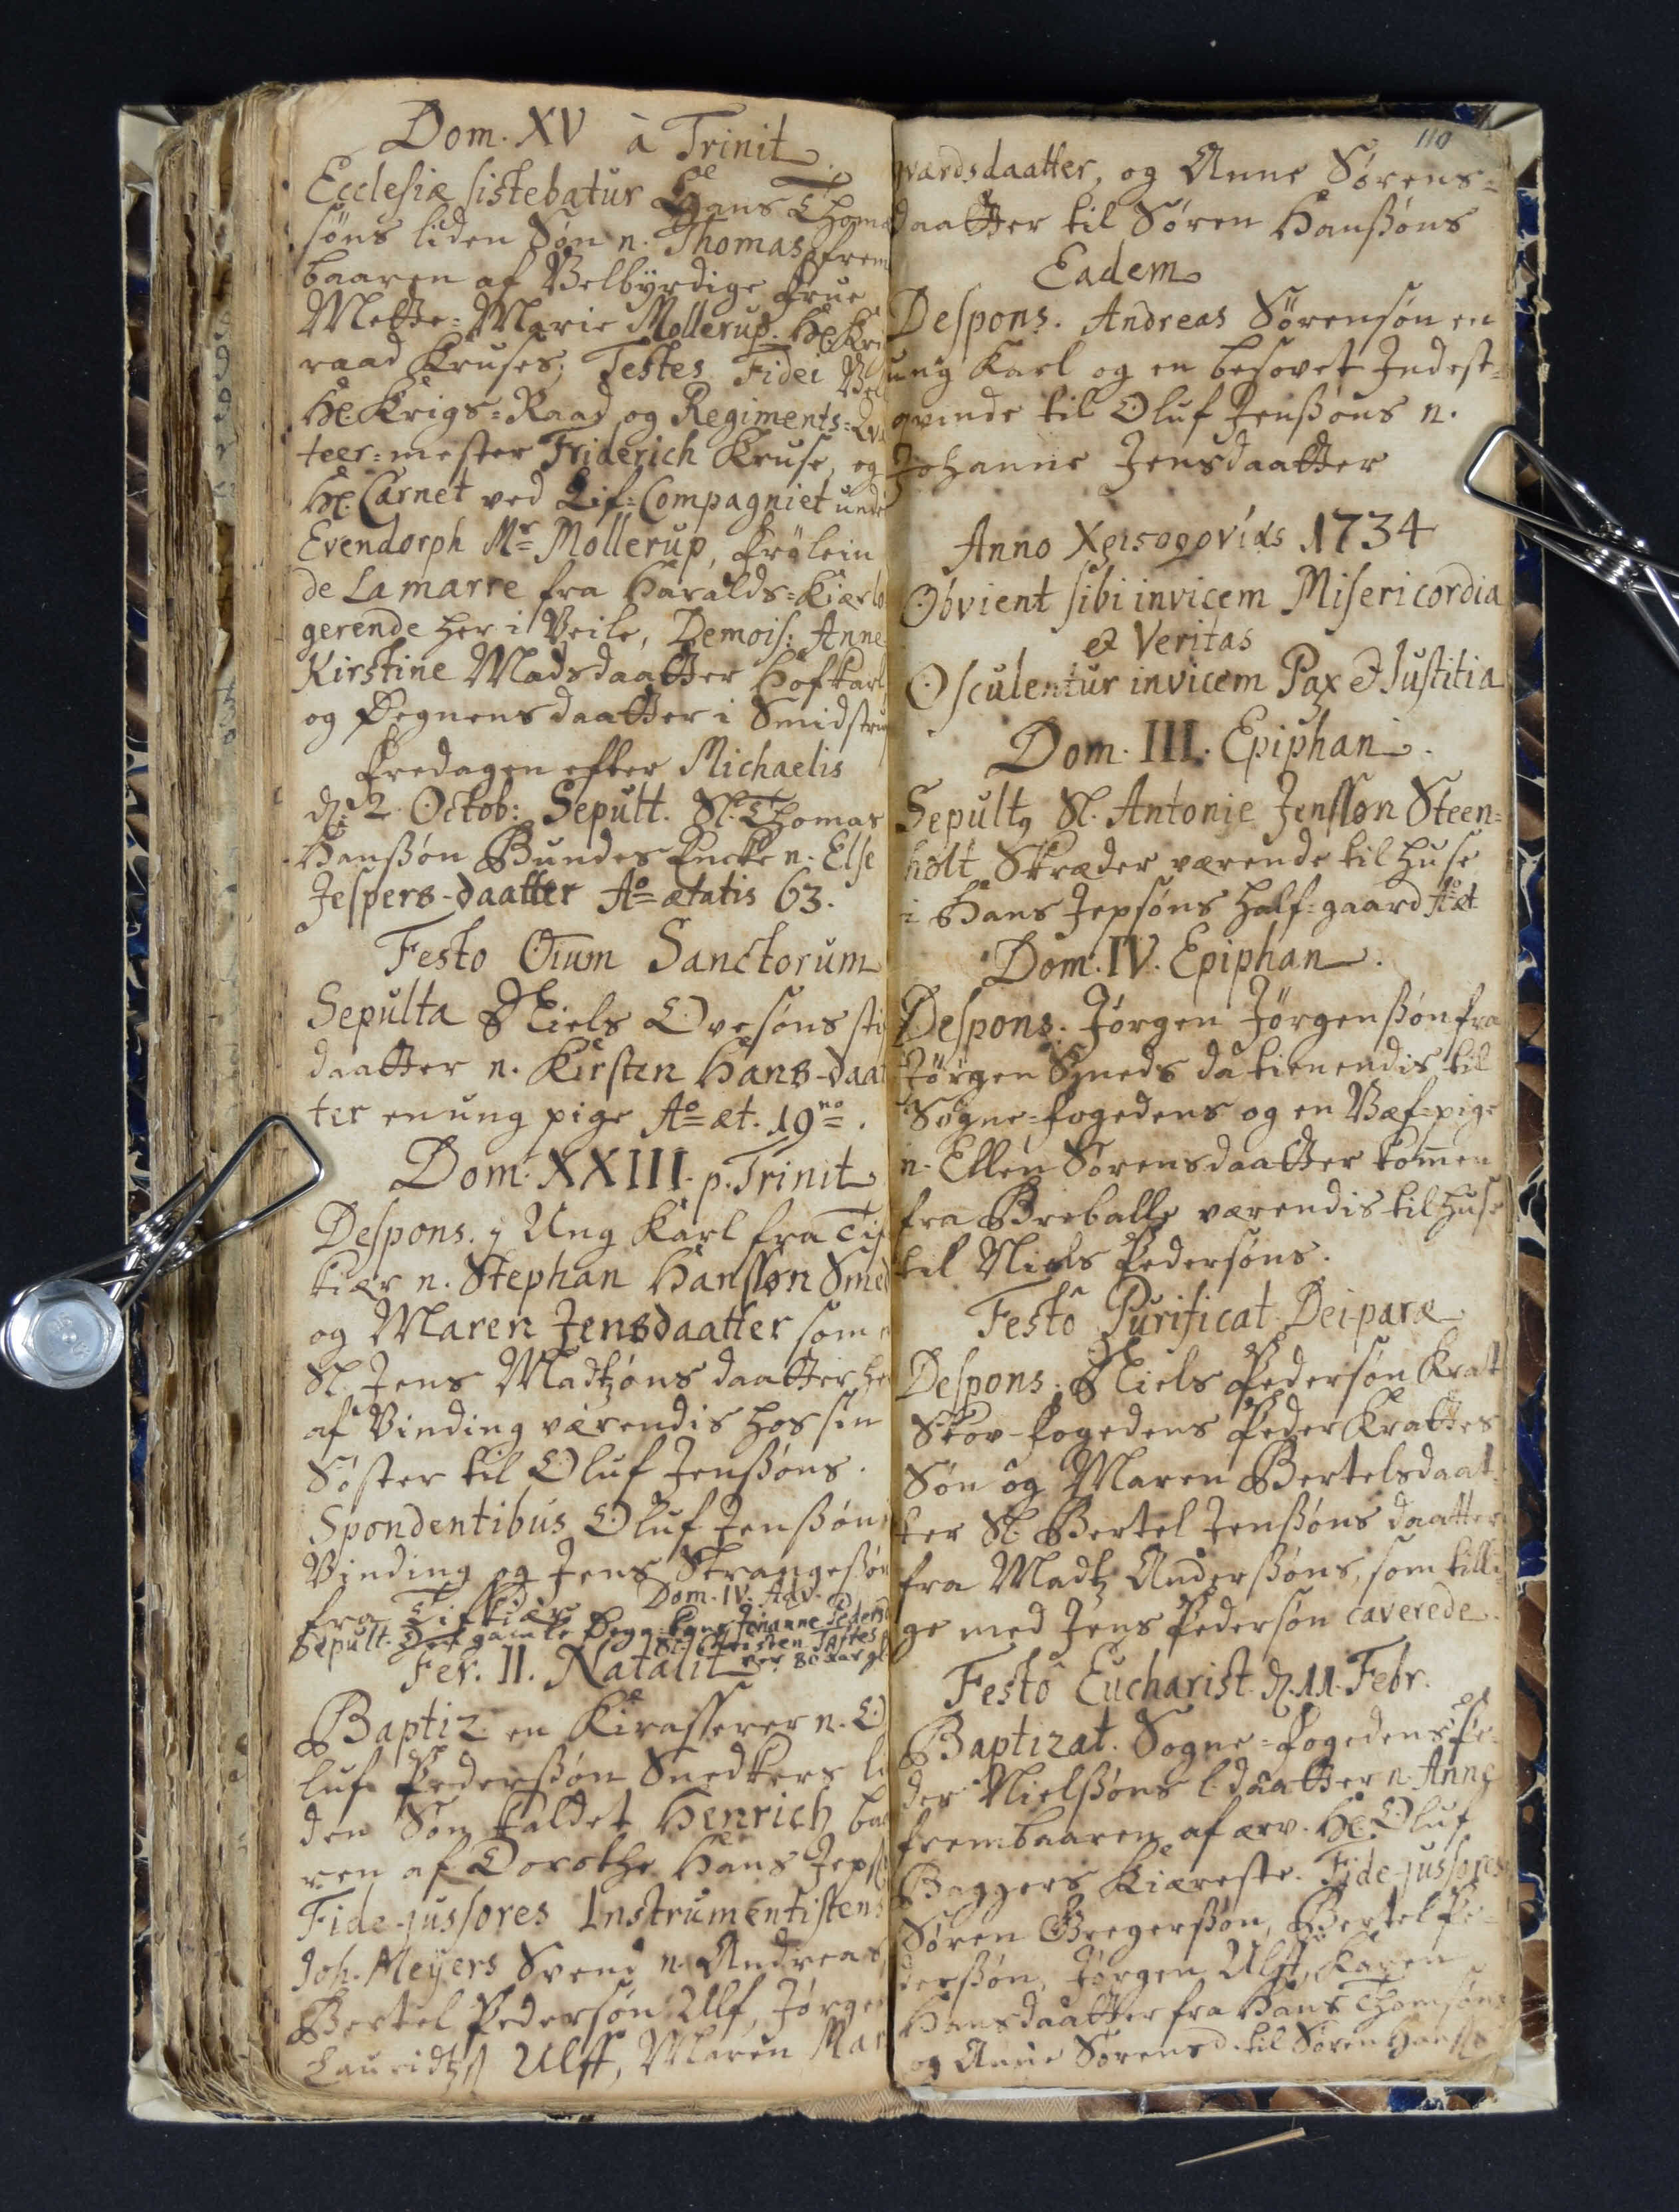Dom. XV a Trinit
Ecclesiæ sistebatur Hans Thomæ
søns liden Søn n. Thomas, frem
baaren af Velbyrdige Frue
Mette=Marie Mollerup Hl Kri  
raad Kruses; Testes Fidei Vel  
Hl Krigs=Raad og Regiments=Qvi  
teer=mester Friderich Kruse, og
Hl Carnet ved Lif=Compagniet unde  
Evendorph Mr Mollerup, Frølein
de la marre fra Haralds=Kiær lo
gerende her i Veile, Demois: Anne
Kirstine Madsdaatter Hofkarl
og Degnens daatter i Smidstrup
Fredagen efter Michaelis
d. 2. Octob: Sepult. Sl. Thomas
Hanssøn Bundes Encke n. Else
Jespers-daatter Ao ætatis 63.
Festo Oium Sanctorum
Sepulta Niels Ovesøns stif
daatter n. Kirsten Hansdaat
ter en ung pige Ao æt. 19no.
Dom. XXIII. p. Trinit
Despons. 1 Ung Karl fra Tif
kiær n. Stephan Hanssøn Smed
og Maren Jensdaatter som
Sl. Jens Madtzøns daatter her  
af Vinding værendis hos sin
Søster til Oluf Jenssøns.
Spondentibus Oluf Jenssøn i
Vinding og Jens Strangessøn
fra Tifkiær.
Dom. IV. Adv.
Sepult. Den gamle Degn=Kone Johanne Pedersd
Sl. Christen Taftes
var 80 aar gl.
Fer. II. Natalit
Baptiz. en Kirasserer n. O
luf Pederssøn Snedkers li
den Søn kaldet Henrich baa  
ren af Dorothe Hans Jeps
Fide-jussores Instrumentistens
Joh. Meyers Svend n. Andreas
Bertel Pedersøn Ulf, Jørgen
Lauridtzs Ulff, Maren Mar
110
qvardsdaatter, og Anne Sørens¬
daatter til Søren Hanssøns
Eadem
Despons. Andreas Sørensøn en
ung Karl og en besovet Indest¬
qvinde til Oluf Jenssøns n.
Johanne Jensdaatter
Anno X##ovixs 1734
obvient sibi invicem Misericordia
et Veritas
Osculentur invicem Pax et Justitia
Dom. III. Epiphan
Sepultq Sl. Antonie Jenssøn Steen¬
holt Skræder værende til huse
i Hans Jepsøns half=gaard Ao æt.
Dom. IV. Epiphan
Despons. Jørgen Jørgenssøn fra
Jørgen Smeds da tienendis til
Sogne=fogedens og en Væf=pige
n. Ellen Sørensdaatter kommen
fra Breballe værendis til huse
til Niels Pedersøns
Festo Purificat Dei-paræ
Despons. Niels Pedersøn Krat
Skov-fogedens Peder Krattes
søn og Maren Bertelsdaat¬
ter Sl. Bertel Jenssøns daatter
fra Madtz Anderssøns som tilli
ge med Jens Pedersøn caverede.
Festo Eucharist d. 11. Febr.
Baptizat. Sogne=Fogedens Pe¬
der Nielssøns l. daatter n. Anne,
frembaaren af ærv. Hl Oluf
Baggers Kiæreste. Fide-jussores
Søren Gregerssøn. Bertel Pe¬
derssøn, Jørgen Ulff, Karen
Hansdaatter fra Hans Thomæsøns
og Anne Sørensd. til Søren Hanss
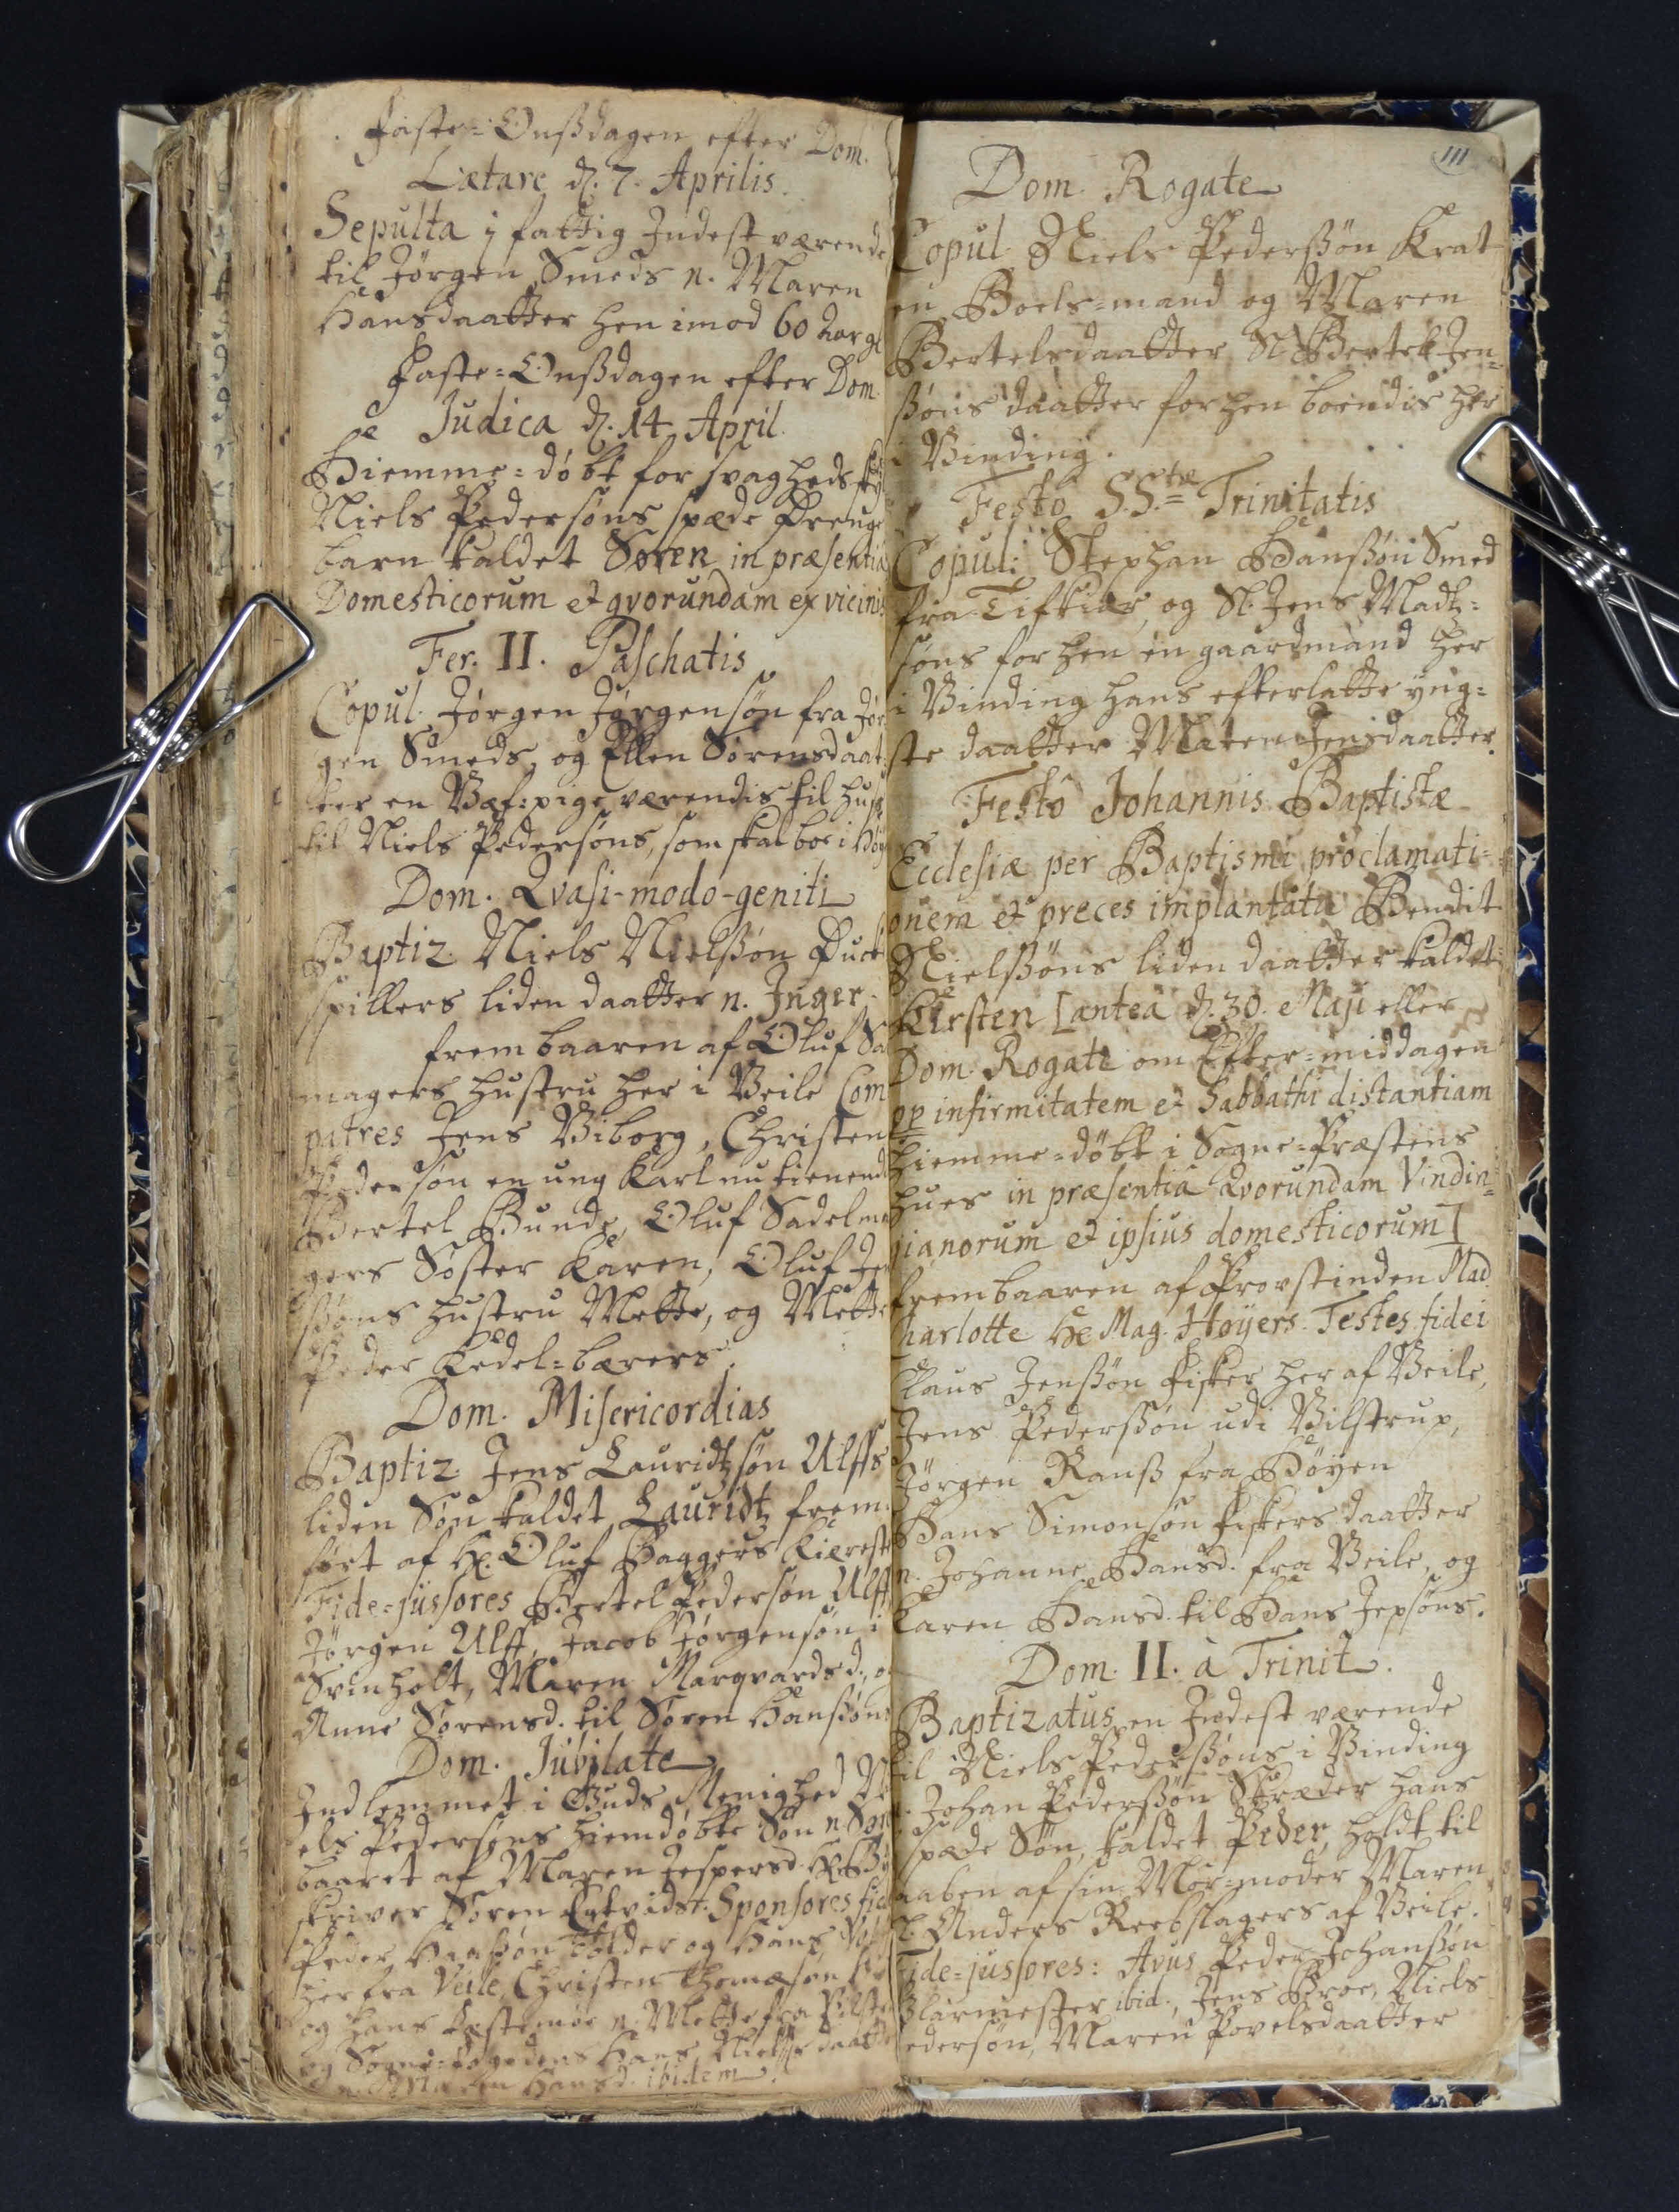Faste=Onssdagen efter Dom.
Lætare d. 7. Aprilis.
Sepulta 1 fattig Indest værende  
til Jørgen Smeds n. Maren
Hansdaatter hen imod 60 Aar gl
Faste=Onssdagen efter Dom
Judica d. 14 April.
Hiemme=døbt for svagheds sky  
Niels Pedersøns spæde Drenge
barn kaldet Søren, in præsentia  
Domesticorum et qvorundam ex vicinis  
Fer. II. Paschatis
Copul. Jørgen Jørgensøn fra Jør¬
gen Smeds, og Ellen Sørensdaat
ter en Væf=pige værendis til huse
til Niels Pedersøns, som skal boe i Høy  
Dom. Qvasi-modo-geniti
Baptiz. Niels Nielssøn Duck  
spillers liden daatter n. Inger,
frembaaren af Oluf Sa  
magers hustru her i Veile Com
patres Jens Viborg, Christen
Pedersøn en ung Karl nu tienende  
Bertel Bunde, Oluf Sadelma 
gers Søster Karen, Oluf Jen  
ssøns hustru Mette, og Mette  
Peder Kedel=bærers
Dom. Misericordias
Baptiz. Jens Lauridtzsøn Ulffs
liden Søn kaldet Lauridtz frem
ført af Hl Oluf Baggers Kiæreste  
Fide=jussors Bertel Pedersøn Ulff  
Jørgen Ulff, Jacob Jørgensøn i
Svinholt, Maren Marqvardsd., og
Anne Sørensd. til Søren Hanssøns
Dom. Jubilate
Indlemmet i Guds Menighed Ni  
els Pedersøns hiemdøbte Søn n. Søn  
baaret af Maren Jespersd. Hl By  
skriver Søren Egtvids. Sponsores
Peder Hanssøn Tolder og Hans
her fra Veile, Christen Thomæsøn
og hans Fæstemøe n. Mette fra
og Sogne=Fogedens Hans Nielss
n. Maren Hansd. ibidem
111
Dom. Rogate.
Copul. Niels Pederssøn Krat
en Boels=mand og Maren
Bertelsdaatter Sl Bertel Jen¬
ssøns daatter for hen boendis her
i Vinding
Festo S.S.tæ Trinitatis
Copul: Stephan Hanssøn Smed
fra Tifkiær og Sl. Jens Madtz¬
søns for hen en gaardmand her
i Vinding hans efterlatte yng¬
ste daatter Maren Jensdaatter
Festo Johannis Baptistæ
Ecclesiæ per Baptismi proclamati¬
onem et preces implantata Bendit 
Nielssøns liden daatter kaldet
Kirsten [antea d. 30 Maji eller
Dom. Rogate om Efter=middagen
pp infirmitatem et Sabbathi distantiam
hiemme=døbt i Sogne præstens
hues in præsentia Qvorundam Vindin¬
qianorum et ipsius domesticorum]
frembaaren af Provstinden Mad.
Charlotte Hl. Mag. Høyers. Testes fidei
Claus Jenssøn Fisker her af Veile.
Jens Pederssøn udi Vilstrup
Jørgen Ranss fra Høyen
Hans Simonsøn Fiskers daatter
n. Johanne Hansd. fra Veile, og
Karen Hansd. til Hans Jepsøns.
Dom. II. a Trinit.
Baptizatus en Indest værende
til Niels Pedersøns i Vinding
Johan Pederssøn Skræder hans
spæde Søn, kaldet Peder, holdt til
 aaben af sin Mor=moder Maren
l. Anders Reebslagers af Veile.
Fide=jussores: Avus Peder Johanssøn
Glarmester ibid., Jens Broe, Niels
edersøn, Maren Povelsdaatter